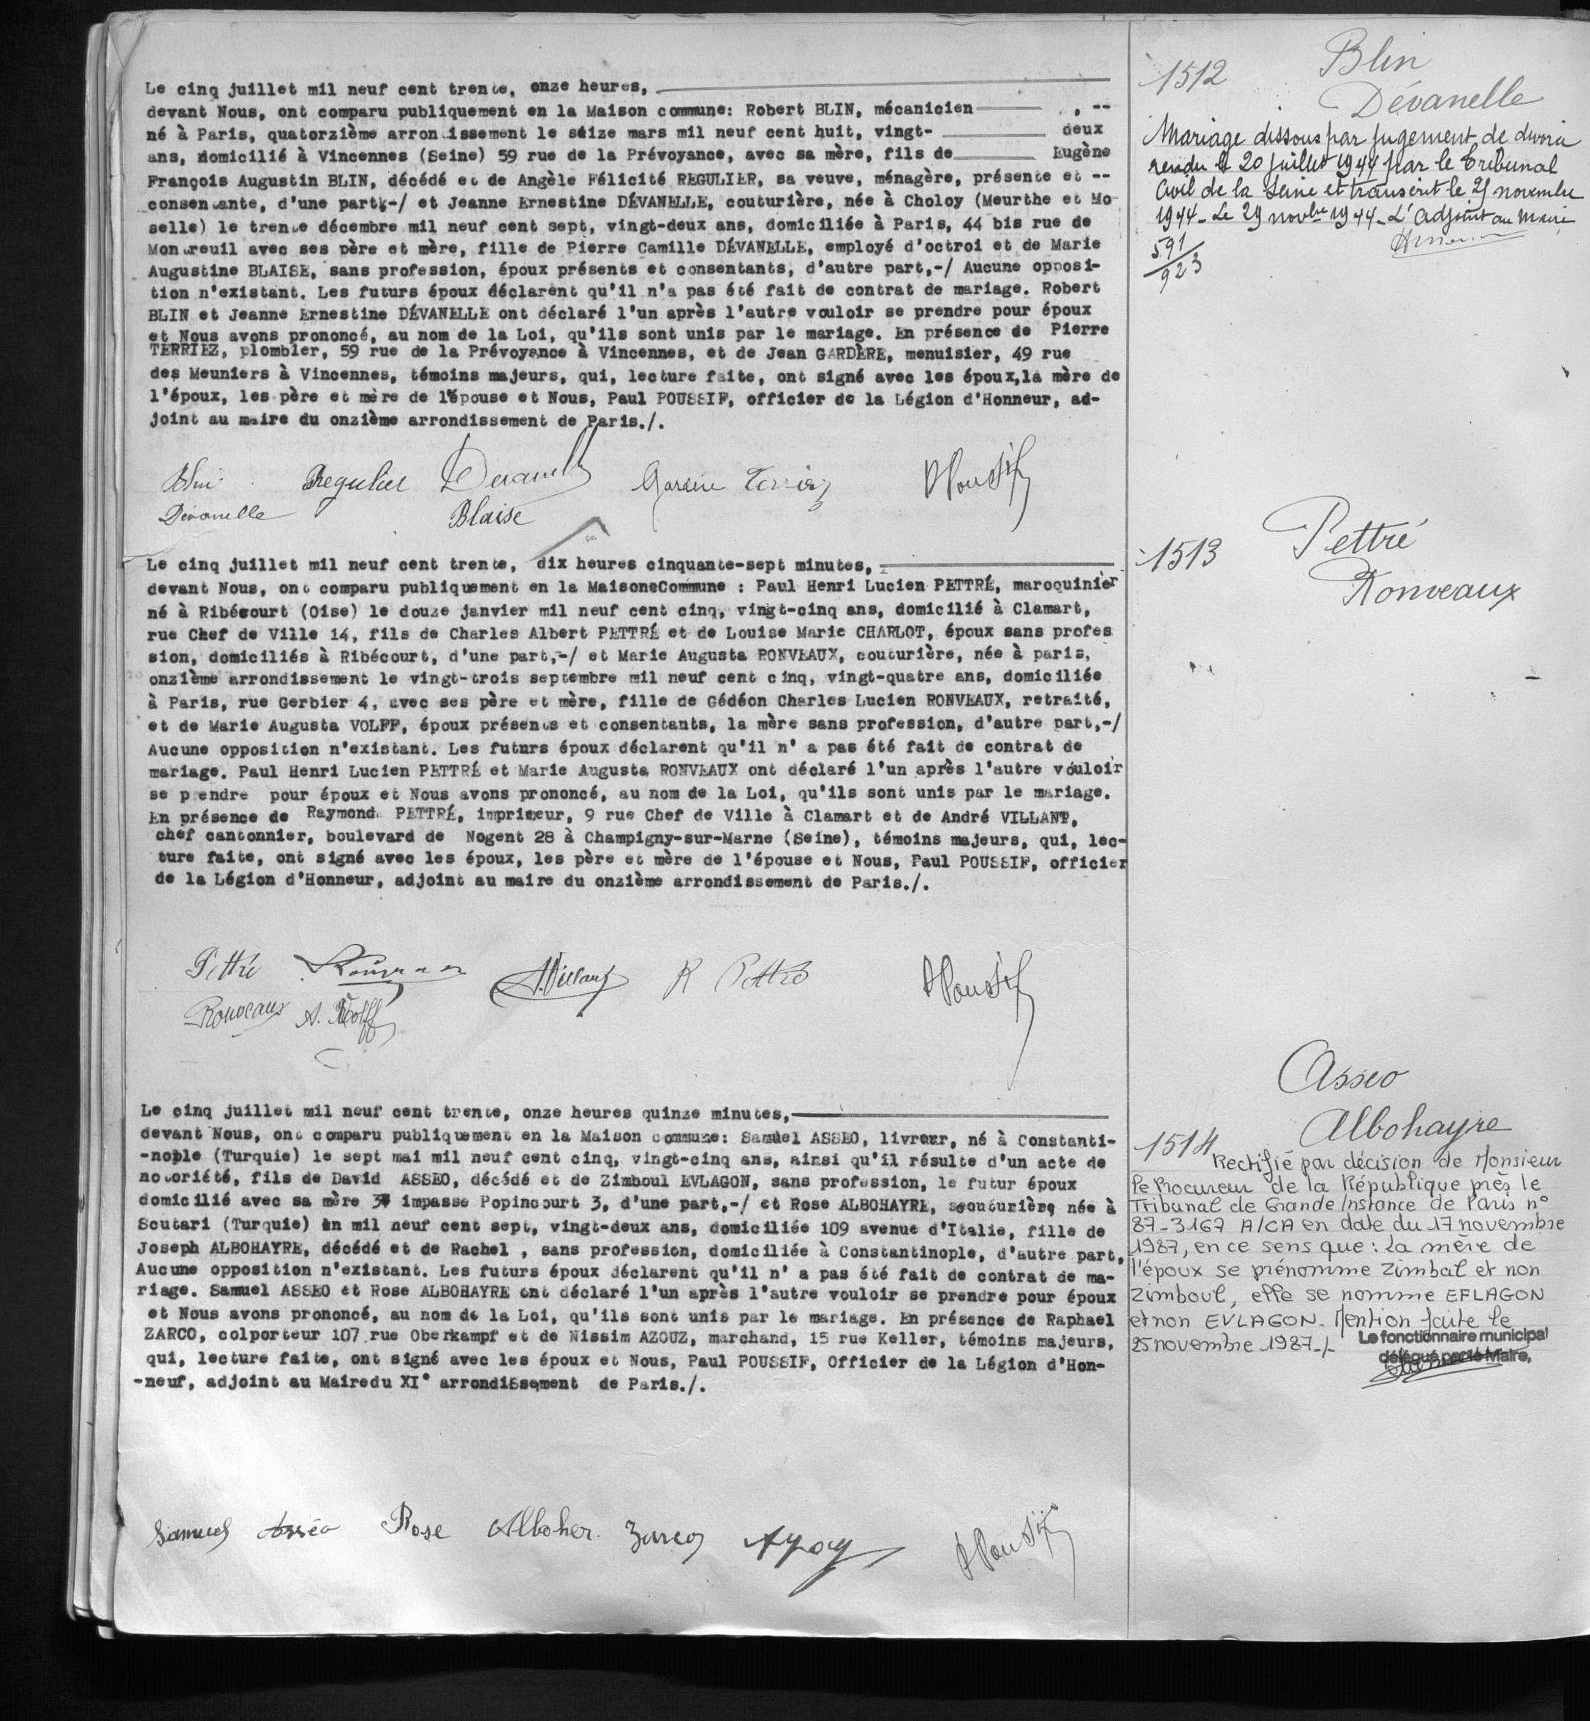Le cinq juillet mil neuf cent trente, onze heures ,-
devant Nous, ont comparu publiquement en la Maison commune: Robert BLIN, mécanicien -, -
né à Paris, quatorzième arrondissement le seize mars mil neuf cent huit, vingt -deux
ans, domicilié à Vincennes (Seine) 59 rue de la Prévoyance, avec sa mère, fils de -Eugène
François Augustin BLIN, décédé et de Angèle Félicité REGULIER, sa veuve, ménagère, présente et -
consentante, d'une part -/ et Jeanne Ernestine DEVANELLE, couturière, née à Choloy (Meurthe et Mo-
selle) le trente décembre mil neuf cent sept, vingt-deux ans, domiciliée à Paris, 44 bis rue de
Mon reuil avec ses père et mère, fille de ierre Camille DEVANELLE, empoyé d'octroi et de Marie
Augustine BLAISE, sans profession, époux présents et consentants, d'autre part ,-/ Aucune opposi-
tion n'existant. Les futurs époux déclerent qu'il n'a pas été fait de contrat de mariage. Robert
BLIN et Jeanne Ernestin DEVANELLE ont déclaré l'un après l'autre vouloir se prendre pour époux
et Nous avons prononcé, au nom de la Loi, qu'ils sont unis par le mariage. En présence de Pierre
TERRIEZ, plombier, 59 rue de la Prévoyance à Vincennes, et de Jean GARDERE, menuisier, 49 rue
des Meuniers à Vincennes, témoins majeurs, qui, lecture faite, ont signé avec les époux, la mère de
l'époux, les père et mère de l'épouse et Nous, Paul POUSSIF, officier de la Légion d'Honneur, ad-
joint au maire du onzième arrondissement de Paris ./.
Le cinq juillet mil neuf cent trente, dix heures cinquante-sept minutes, -
devant Nous, ont comparu publiquement en la Maison * Commune: Paul Henri Lucien PETTRE, maroquinier
né à Ribésourt (Oise) le douze janvier mil neuf cent cinq, vingt-cinq ans, domiciliée à Clamart,
rue Chef de Ville 14, fils de Charles Albert PETTRE et de Louis Marie CHARLOT, époux sans profes
sion, domiciliés à Ribécourt, d'une part, -/ et Marie Augusta RONAVEAUX, couturière, née à paris,
onzième arrondissement le vingt-trois septembre mil neuf cent cinq, vingt-quatre ans, domiciliée
à Paris, rue Gerbier 4, avec ses père et mère, fille de Gédéon Charles Lucien RONVEAUX, retraité,
et de Marie Augusta VOLFF, époux présents et consentants, la mère sans profession, d'autre part ,-/
Aucune opposition n'existant. Les futurs époux déclarent qu'il n'a pas été fait de contrat de
mariage. Paul Henri Lucien PETTRE et Marie Augusta RONVEAUX ont déclaré l'un après l'autre vouloir
se prendre pour époux et Nous avons prononcé, au nom de la Loi, qu'ils sont unis par le mariage.
En présence de Raymond PETTRE, imprimeur, 9 rue Chef de Ville à Clamart et de André VILLANT,
chef cantonnier, boulevard de Nogent 28 à Champigny-sur-Marne (Seine), témoins majeurs, qui, lec-
ture faite, ont signé avec les époux, les père et mère de l'épouse et Nous, Paul POUSSIF, officier
de la Légion d'Honneur, adjoint au maire du onzième arrondissement de Paris ./.
Le cinq juillet mil neuf cent trente, onze heures quinze minutes, -
devant Nous, ont comparu publiquement en la Maison commune: Samuel ASSEO, livreur, née à Constanti-
-nople (Turquie) le sept mai mil neuf cent cinq, vingt-cinq ans, ainsi qu'il résulte d'un acte de
notoriété, fils de David ADDEO, décédé et de Zimboul EVLAGON, sans profession, le futurs époux
domicilié avec sa mère 38 impasse Popincourt 3, d'une part ,-/ et Rose ALBOHAYRE, couturière, née à
Soutari (Turquie) en mil neuf cent sept, vingt-deux ans, domiciliée 109 avenue d'Italie, fille de
Joseph ALBOHAYRE, décédé et de Rachel, sans profession, domiciliée à Constantinople, d'autre part,
Aucune opposition n'existant. Les futurs époux déclarent qu'il n'a pas été fait de contrat de ma-
riage. Samuel ASSEO et Rose ALBOHAYRE ont déclaré l'un après l'autre vouloir se prendre pour époux
et Nous avons prononcé, au nom de la Loi, qu'ils sont unis par le mariage. En présence de Raphael
ZARCO, colporateur 107 rue Oberkampf et de Nissim AZOUZ, marchand, 15 rue Keller, témoins majeurs,
qui, lecture faite, ont signé avec les époux et Nous, Paul POUSSIF, Officier de la Légion d'Hon-
-neuf, adjoint au Maire du XI ° arrondiSsement de Paris ./.
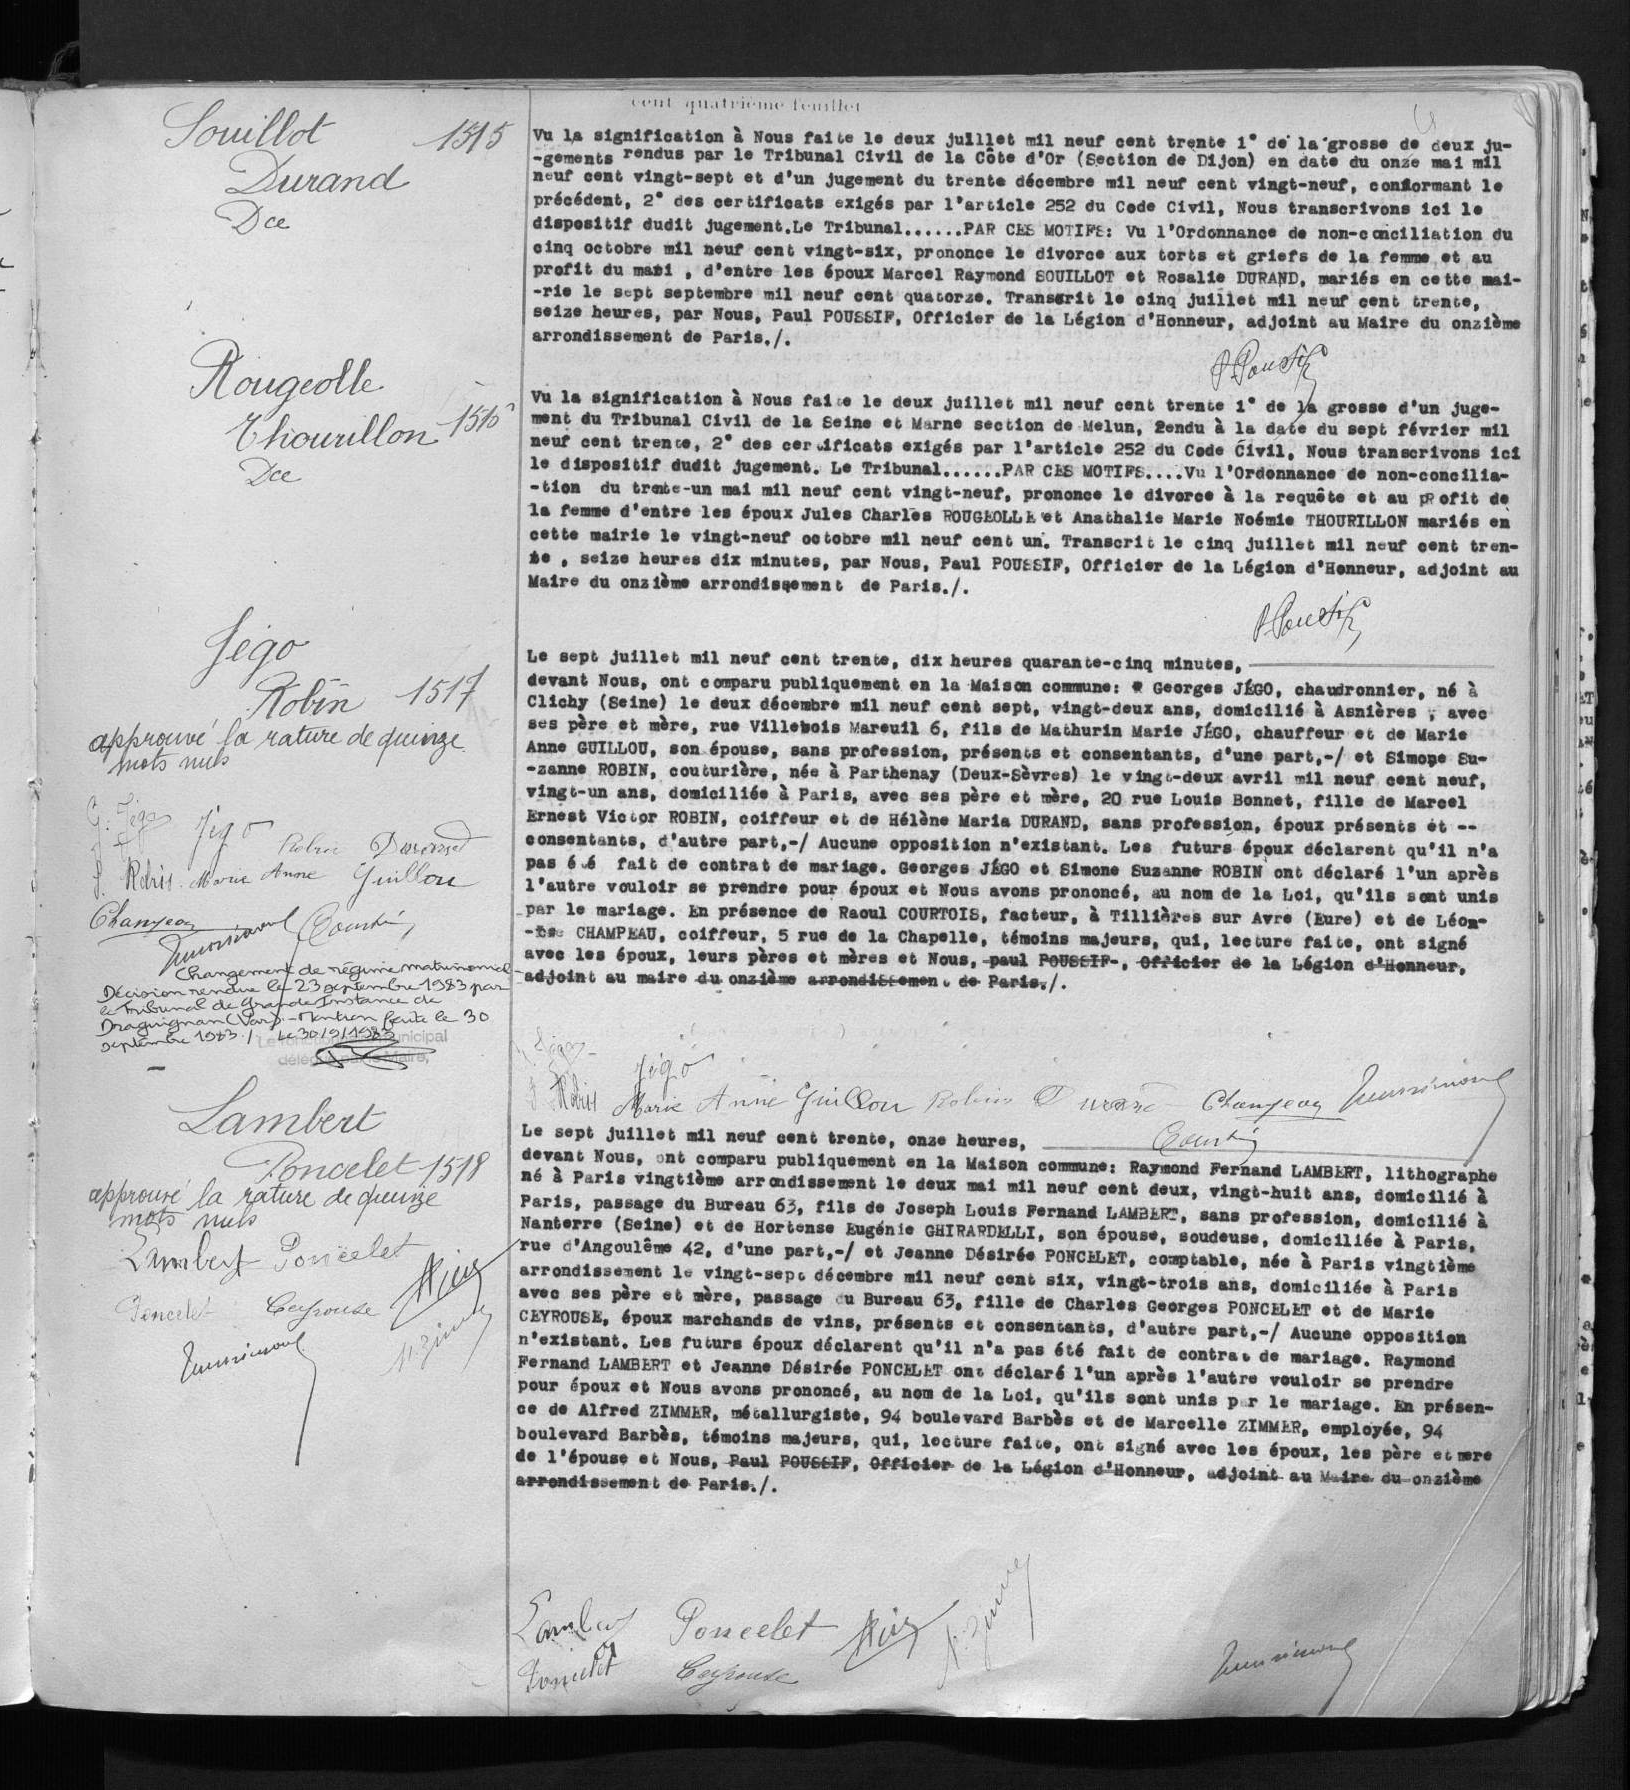Vu la signification à Nous faite le deux juillet mil neuf cent trente 1° de la grosse de deux ju-
gements rendu par le Tribunal Civil de la Côte d'Or (Section de Dijon) en date du onze mai mil
neuf cent vingt-sept et d'un jugement du trente décembre mil neuf cent vingt-neuf, conformant le
précédent, 2° des certificats exigés par l'article 252 du Code Civil, Nous transcrivons ici le
dispositif dudit jugement. Le Tribunal... PAR CES MOTIFS: Vu l'Ordonnance de non-conciliation du
cinq octobre mil neuf cent vingt-six, prononce le divorce aux torts et griefs de la femme et au
profit du mari, d'entre les époux Marcel Raymon SOUILLOT et Rosalie DURAND, mariés en cette mai-
-rie le sept septembre mil neuf cent quatorze. Transcrit le cinq juillet mil neuf cent trente,
seize heures, par Nous, Paul POUSSIF, Officier de la Légion d'Honneur, adjoint au Maire du onzième
arrondissement de Paris ./.
Vu la signification à Nous faite le deux juillet mil neuf cent trente 1° de la grosse d'une juge-
ment du Tribunal Civil de la Seine et Marne section de Melun, 2endu à la date du sept février mil
neuf cent trente, 2° des certificats exigés par l'article 252 du Code Civil, Nous transcrivons ici
le dispositif dudit jugement. Le Tribunal..... PAR CES MOTIFS.... Vu l'Ordonnance de non-concilia-
-tion du trente-un mai mil neuf cent vingt-neuf, prononce le divorce à la requête et au pRofit de
la femme d'entre les époux Jules CHarles ROUGEOLLE et Anathalie Marie Noémie THOURILLON mariés en
cette mairie le vingt-neuf octobre mil neuf cent un. Transcrit le cinq juillet mil neuf cent tren-
te, seize heures di minutes, par nous, Paul POUSSIF, Officier de la Légion d'Honneur, adjoint au
Maire du onzième arrondissement de Paris ./.
Le sept juillet mil neuf cent trente, dix heures quarante-cinq minutes, -
devant Nous, ont comparu publiquement en la Maison commune: * Georges JEGO, chaudronnier, né à
Clichy (Seine) le deux décembre mil neuf cent sept, vingt-deux ans, domicilié à Asnières, avec
ses père et mère, rue Villebois Mareuil 6, fils de Mathurin Marie JEGO, chauffeur et de Marie
Anne GUILLOU, son épouse, sans profession, présents et ocnsentants, d'une part ,-/ et Simone Su-
-zanne ROBIN, couturière, née à Parthenay (Deux-Sèvres) le vingt-deux avril mil neuf cent neuf,
vingt-un ans, domiciliée à Paris, avec ses père et mère, 20 rue Louis Bonnet, fille de Marcel
Ernest Victor ROBIN, coiffeur et de Hélène Maria DURAND, sans profession, époux présents et-
consentants, d'autre part, -/ Aucune opposition n'exisant. Les futurs époux déclarent qu'il n'a
pas été fait de contrat de mariage. Georges JEGO et Simone Suzanne ROBIN ont déclaré l'un après
l'autre vouloir se prendre pour époux et nous avons prononcé, au nom de la Loi, qu'ils sont unis
par le mariage. En présence de Raoul COURTOIS, facteur, à Tillières sur Avre (Eure) et de Léon-
-ce CHAMPEAU, coiffeur, 5 rue de la Chapelle, témoins majeurs, qui, lecture faite, ont signé
avec les époux, leurs père et mère et Nous, paul POUSSIF, Officier de la Légion d'Honneur,
adjoint au maire du onzième arrondissemen de Paris ./.
Le sept juillet mil neuf cent trente, onze heures, -
devant Nous, ont comparu publiquement en la Maison commune: Raymond Fernand LAMBERT, lithographe
né à Paris vingtième arrondissement le deux mai mil neuf cent deux, vingt-huit ans, domicilié à
Paris, passage du Bureau 63, fils de Joseph Louis Fernand LAMBERT, sans profession, domicilié à
Nanterre (Seine) et de Hortense Eugénie GHIRARDELLI, son épouse, soudeuse, domiciliée à Paris,
rue d'Angoulême 42, d'une part ,-/ et Jeanne Désiré PONCELET, comptable, née à Paris vingtième
arrondissement le vingt-sept décembre mil neuf cent six, vingt-trois ans, domiciliée à Paris
avec ses père et mère, passage du Bureau 63, fille de Charles Georges PONCELET et de Marie
CEYROUSE, époux marchands de vins, présents et consentants, d'autre part, -/ Aucune opposition
n'existant. Les futurs époux déclarent qu'il n'a pas été fait de contrat de mariage. Raymond
Fernand LAMBERT et Jeanne Désirée PONCELET ont déclaré l'un après l'autre vouloir se prendre
pour époux et Nous avons prononcé, au nom de la loi, qu'ils sont unis par le mariage. En présen-
ce de Alfred ZIMMER, métallurgiste, 94 boulevard Barbès et de Marcel ZIMMER, employée, 94
boulevard Barbès, témoins majeurs, qui, lecture faite, ont signé avec les époux, les père et mere
de l'épouse de nous, Paul POUSSIF, Officier de la Légion d'Honneur, adjoint au Maire du onzième
arrondissement de Paris ./.